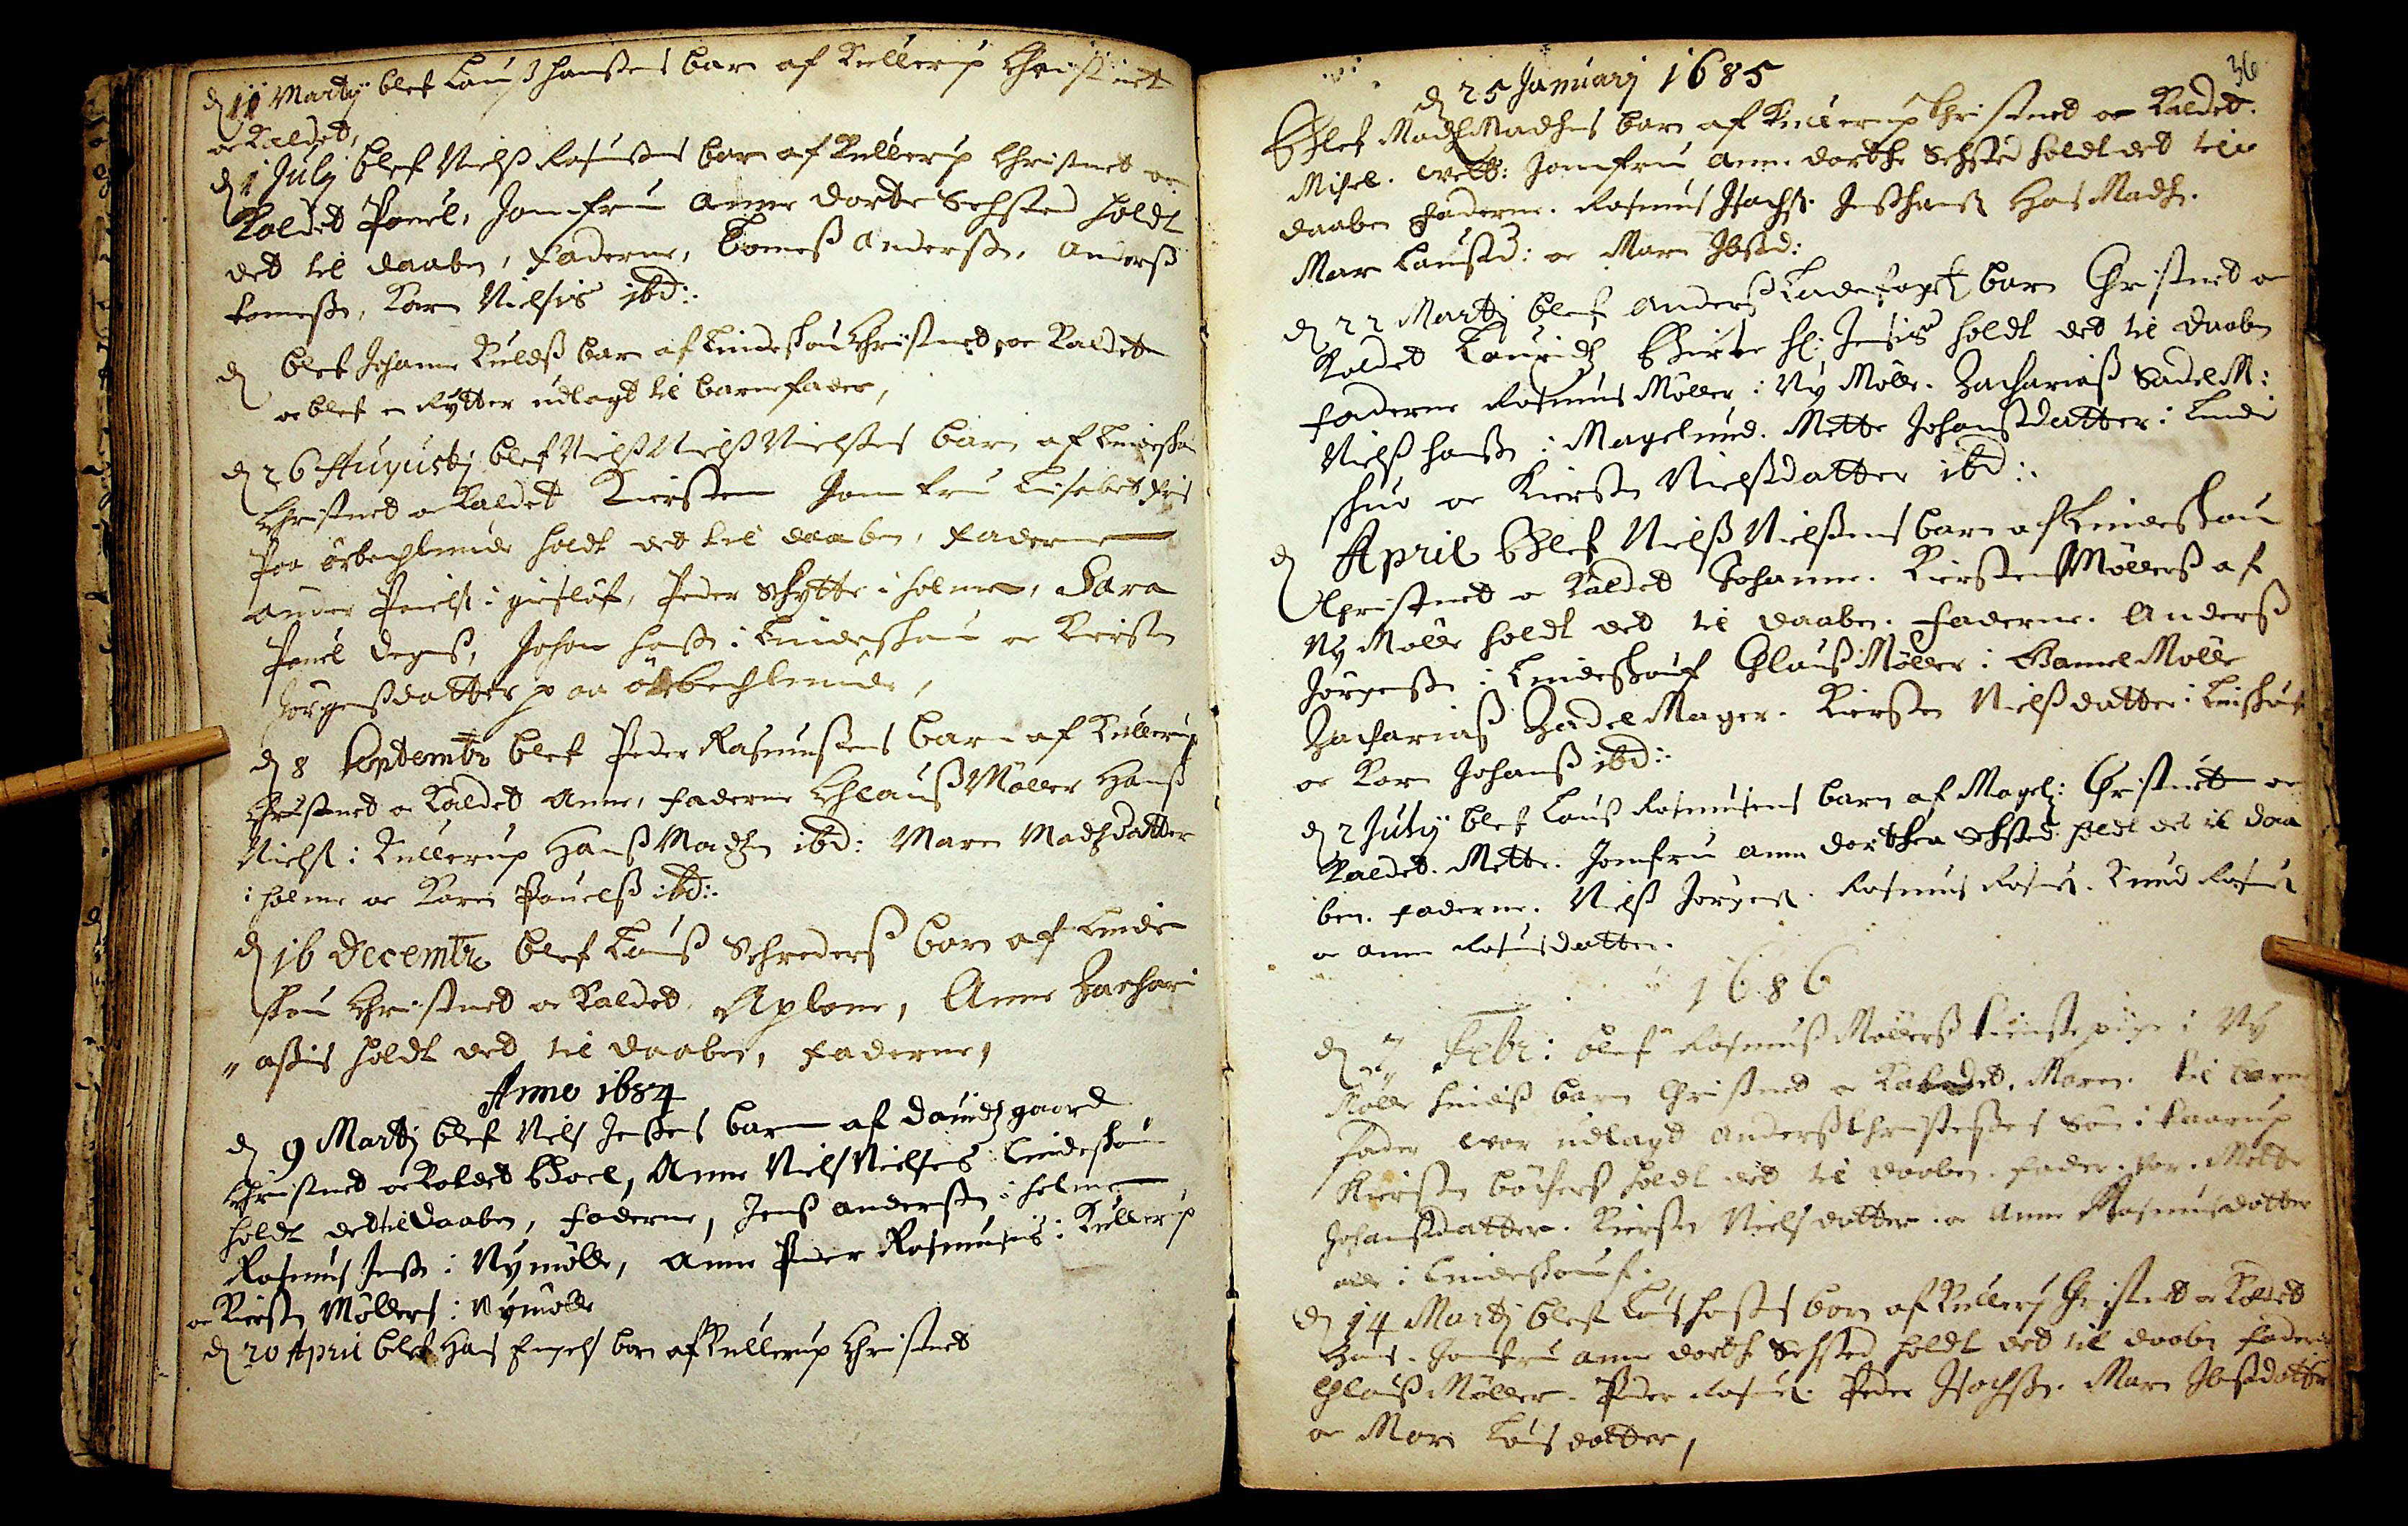d 11 Martij blef Lauss Hanssens barn af Kullerup Christinet
or kaldet,
d 1 Julij blef Niels Rasmussens barn af Kullerup Christnet oc
kaldet Pouel, Jomfru Anne Dorte Sehsted holdt
det til daaben, Faderne, Tomess anderss. Anderss
tommess, Karen Nielsis ibd:.
d blef Johanne Kiuldss barn af Lindeskou Christnet oc Kaldet
oc blef en Rytter udlagt til barnefader,
d 26 Augustij bleff Niels Niels Nielsen barn af Lindeskou
Christnet oc kaldet Kiersten Jomfru Lisabet ##
fra ørbechlund holdt det til daaben, Faderene
Anders Poulss i giesløf, Peder Skytte i holme, Sara
Pouel Degens, Johan Hanss i Lindeskoun oc Kirsten
Jørgensdatter paa ørbechlund
d 8 Septembr blef Peder Rasmussens Barn aff Kullerup
Christned oc kaldet Anne, Faderne Chlauss Møller Hanss
Niels i Kullerup Hanss Madtzen ibd: Maren Madtzdatter
i holme oc Karen Pouels ibd:
d 16 Decembr blef Lauss Schreders barn af Linde
skou Christned oc kaldet Aplone, Anne Zachari
=asis holdt ded til daaben, Faderne,
Anno 1684
d 9 Martij blef Nels Jesens bar af Dauidtzgaard.
Christned oc kaldet Boel, Anne Niels Nielses i Lindeskou
holdt det til daaben, Faderne, Jenss Andersen i Holme
Rasmus Jenss i Nymølle, Anne Peder Rasmusis i Kullerup
oc Kiersten Møllers i Nymølle
d 20 April blef Hans ##Engels barn af Kullerup Christenet
36
d 25 Januarij 1685
Blef Mads Madsens barn af Kullerup Christnet oc kaldet
Misel. Wetb: Jomfru Anne Dorthe Sehsted holdt det till
daaben Faderne. Rasmus Isachss. Jens Hans Hans Mads
Marn Laussd: oc Marn Jbssd:
d 22 Martij blef Anderss Ladefogets barn Christnet oc
kaldet Lauridtz Birte hl: Jensis holdt det til Daabn
Faderne Rasmus Møller i Ny Mølle. Zacharias Sadel M:
Niels Hanss i Magelund. Mette Johansdatter i Linde
skuo oc Kiersten Nielsdatter ibd:.
d Apnil Blef Nielss Nielssens barn af Lindeskou
Christnet oc kaldet Johanne. Kirsten Møllerss af
Ny Mølle holdt det til Daaben. Faderne. Anderss
Jørgenssen i Lindeskouf Chlauss Møller i Gamel Mølle
Zachariass Zadel Mager. Kiersten Nielsdatter i Linskouf
oc Karen Johanss ibd:.
d 2 Julij blef Lauss Rasmussens barn af Magel: Christnet oc
kaldet Mette. Jomfru Anne dorthen Sehsted foldt det til daa
ben. Faderne. Niels Jørgens Rasmus Rasmus Knud Rasmus
oc Anne Rasmusdatter.
1686
d 7 Febr: blef Røsmuss Møllerss Tienstepige i Ny
Mølle hindeis barn Christnet oc kaldet, Maren til barn
fader var udlagt Anderss Christenssens Søn i Taarup
Kiersten Bøcherss holdt det til daaben. Fader. var Mette
Johansdatter. Kiersten Nielsdatter oc Anne Rasmusdatter
alle i Lindeskouf.
d 14 Martij blef Laus hostes barn af Kullerup Christnet oc Koldet
Hans. Jomfru Anne Dorth Sehsted holdt det til daaben Faderne
Chlauss Møller. Peder Rasmus Peder Isachsen. Maren Ibssdatter
oc Maren Lausdatter,
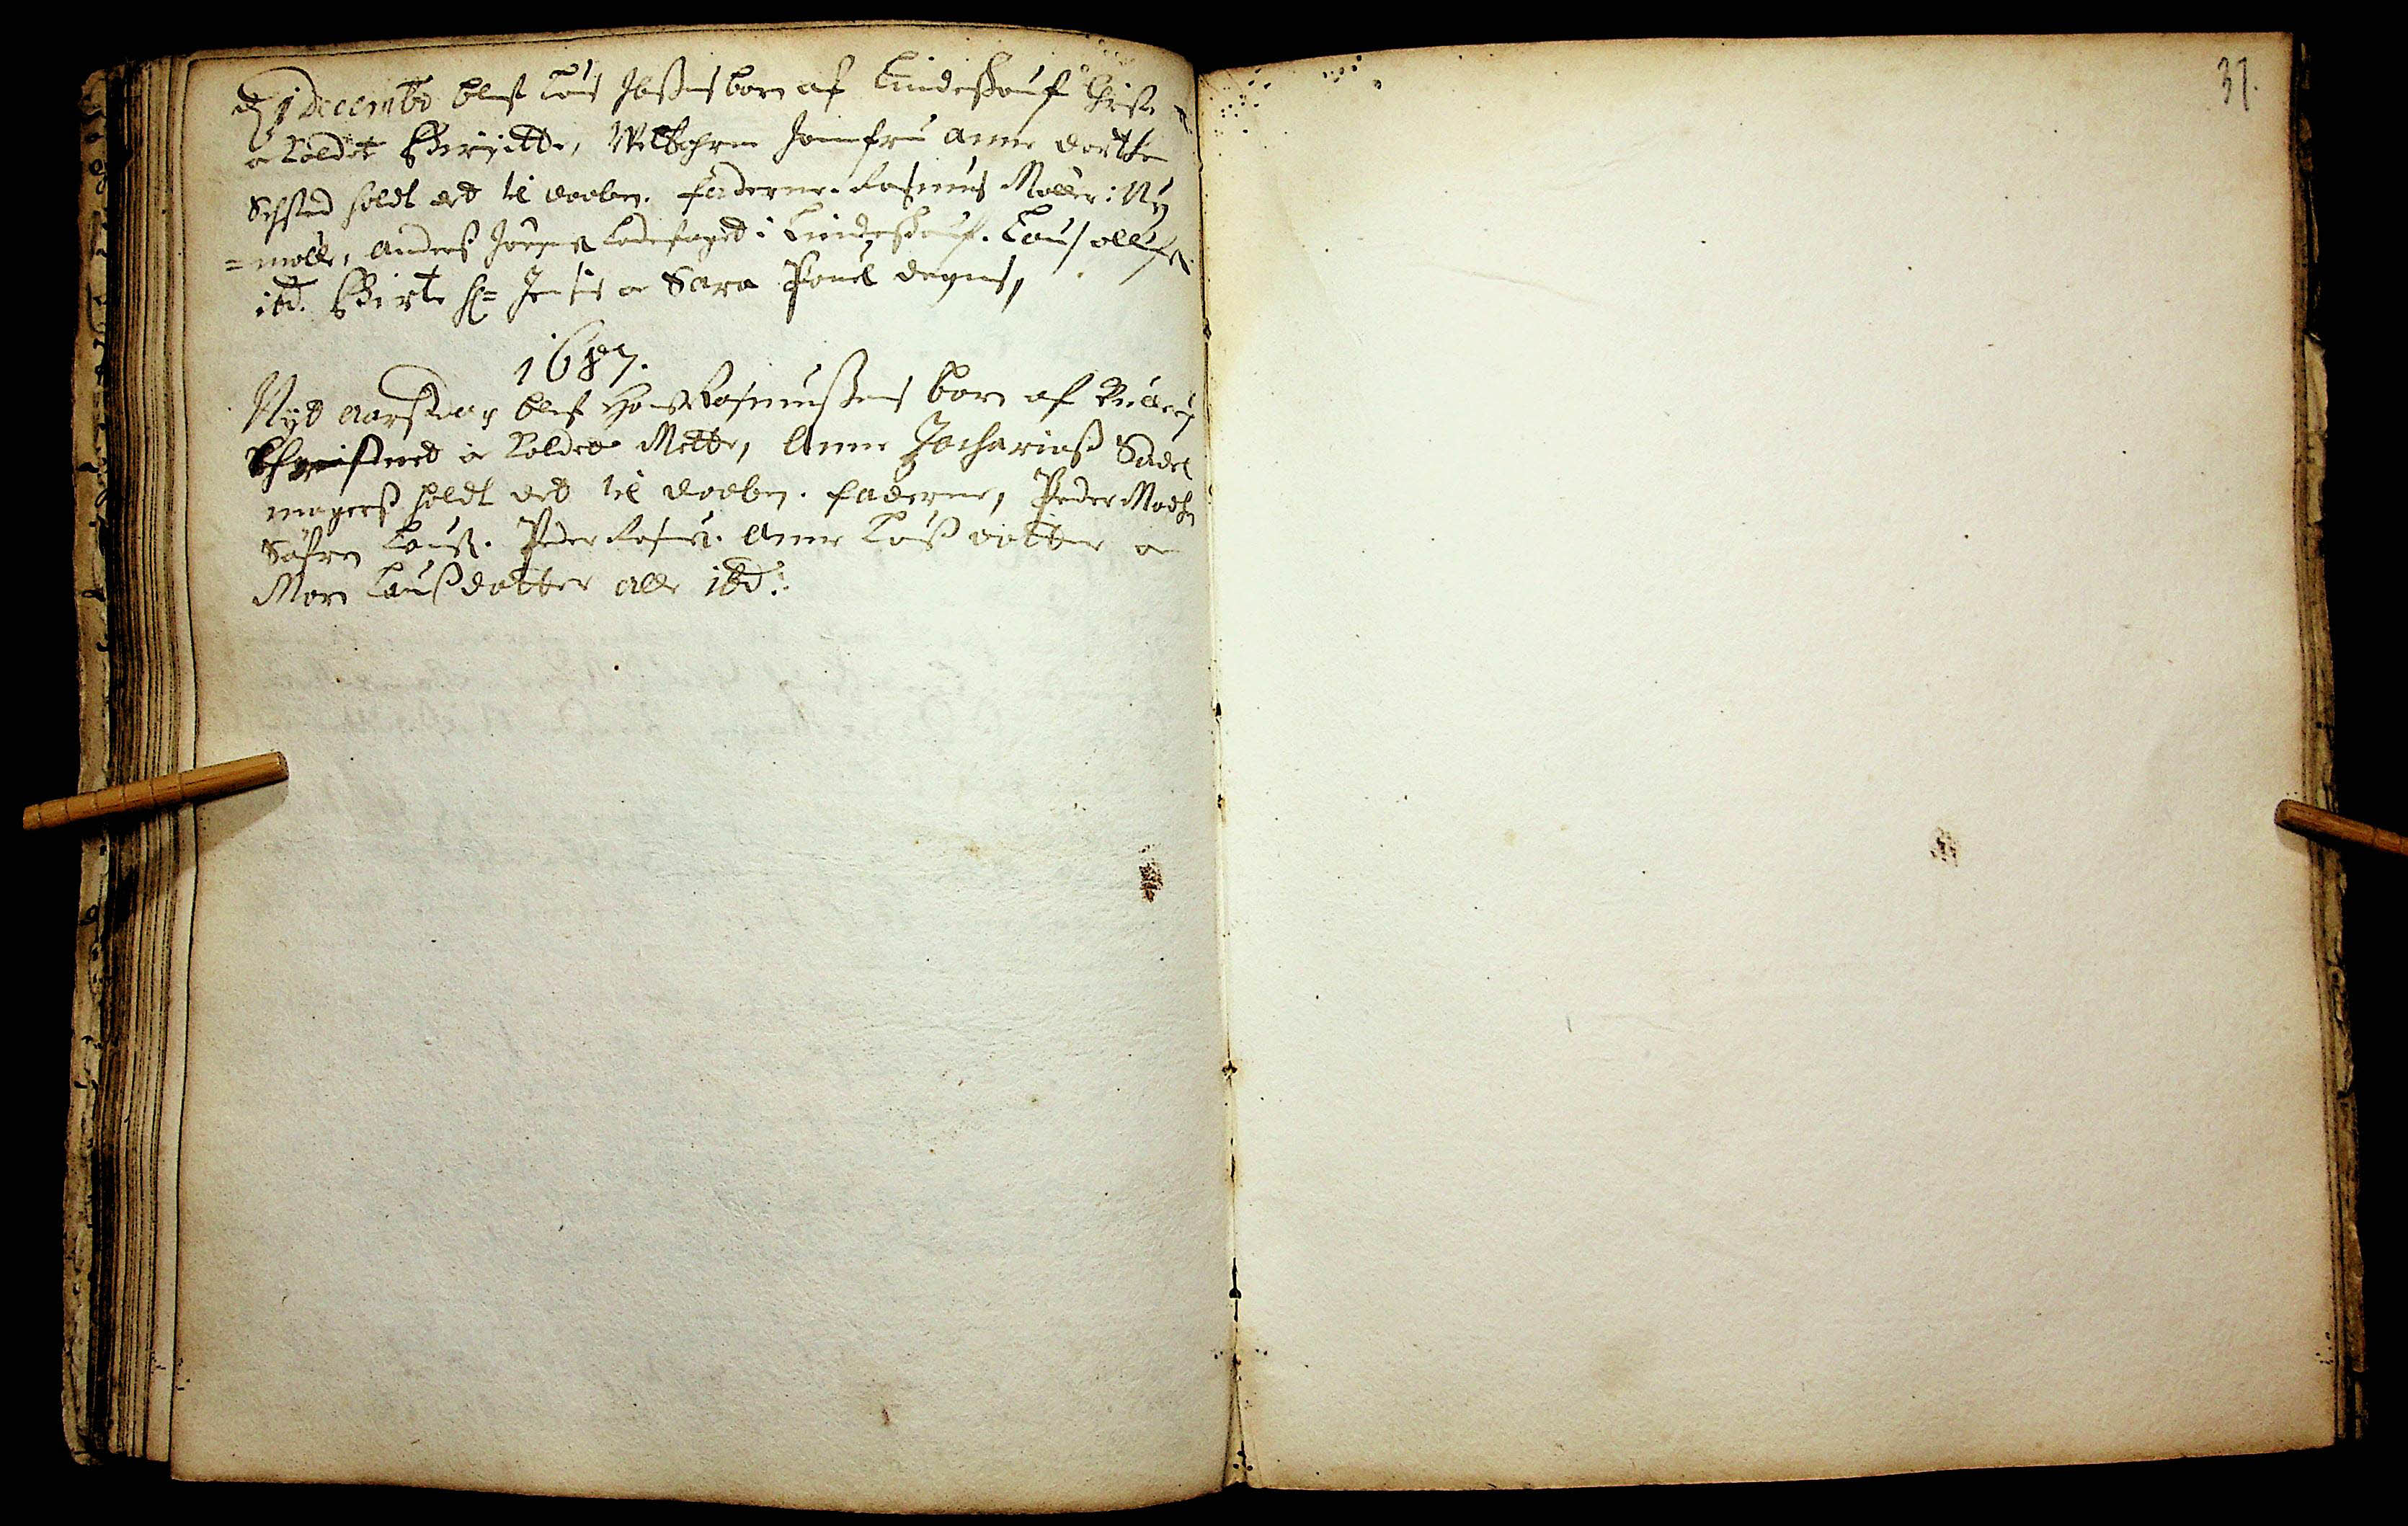d 1 Decembr blef Laus Ibssens barn af Lindeskouf Christ
oc kaldet Birgitte Welbohrne Jomfru Anne Dorthe
Sehsted holdt det til Daaben. Fadderen Rasmus Møller i Ny
= Mølle, Anderss Jørgens Ladefoget i Lindeskouf. Laus ollufs
ibd. Birte hl= Jensis oc Sara Pouel Degns,
1687
Nyt Aarssdag blef Hanss Rasmussens barn af Kullerup
Christnet oc Kaldet Mette, Anne Jachriass Sadel
magerss holdt det til daaben. Faderne, Peder Mads
Søfren Lausss. Peder Rasmus. Anne Lausaatter oc
Marn Lausdatter alle ibd:.
31.